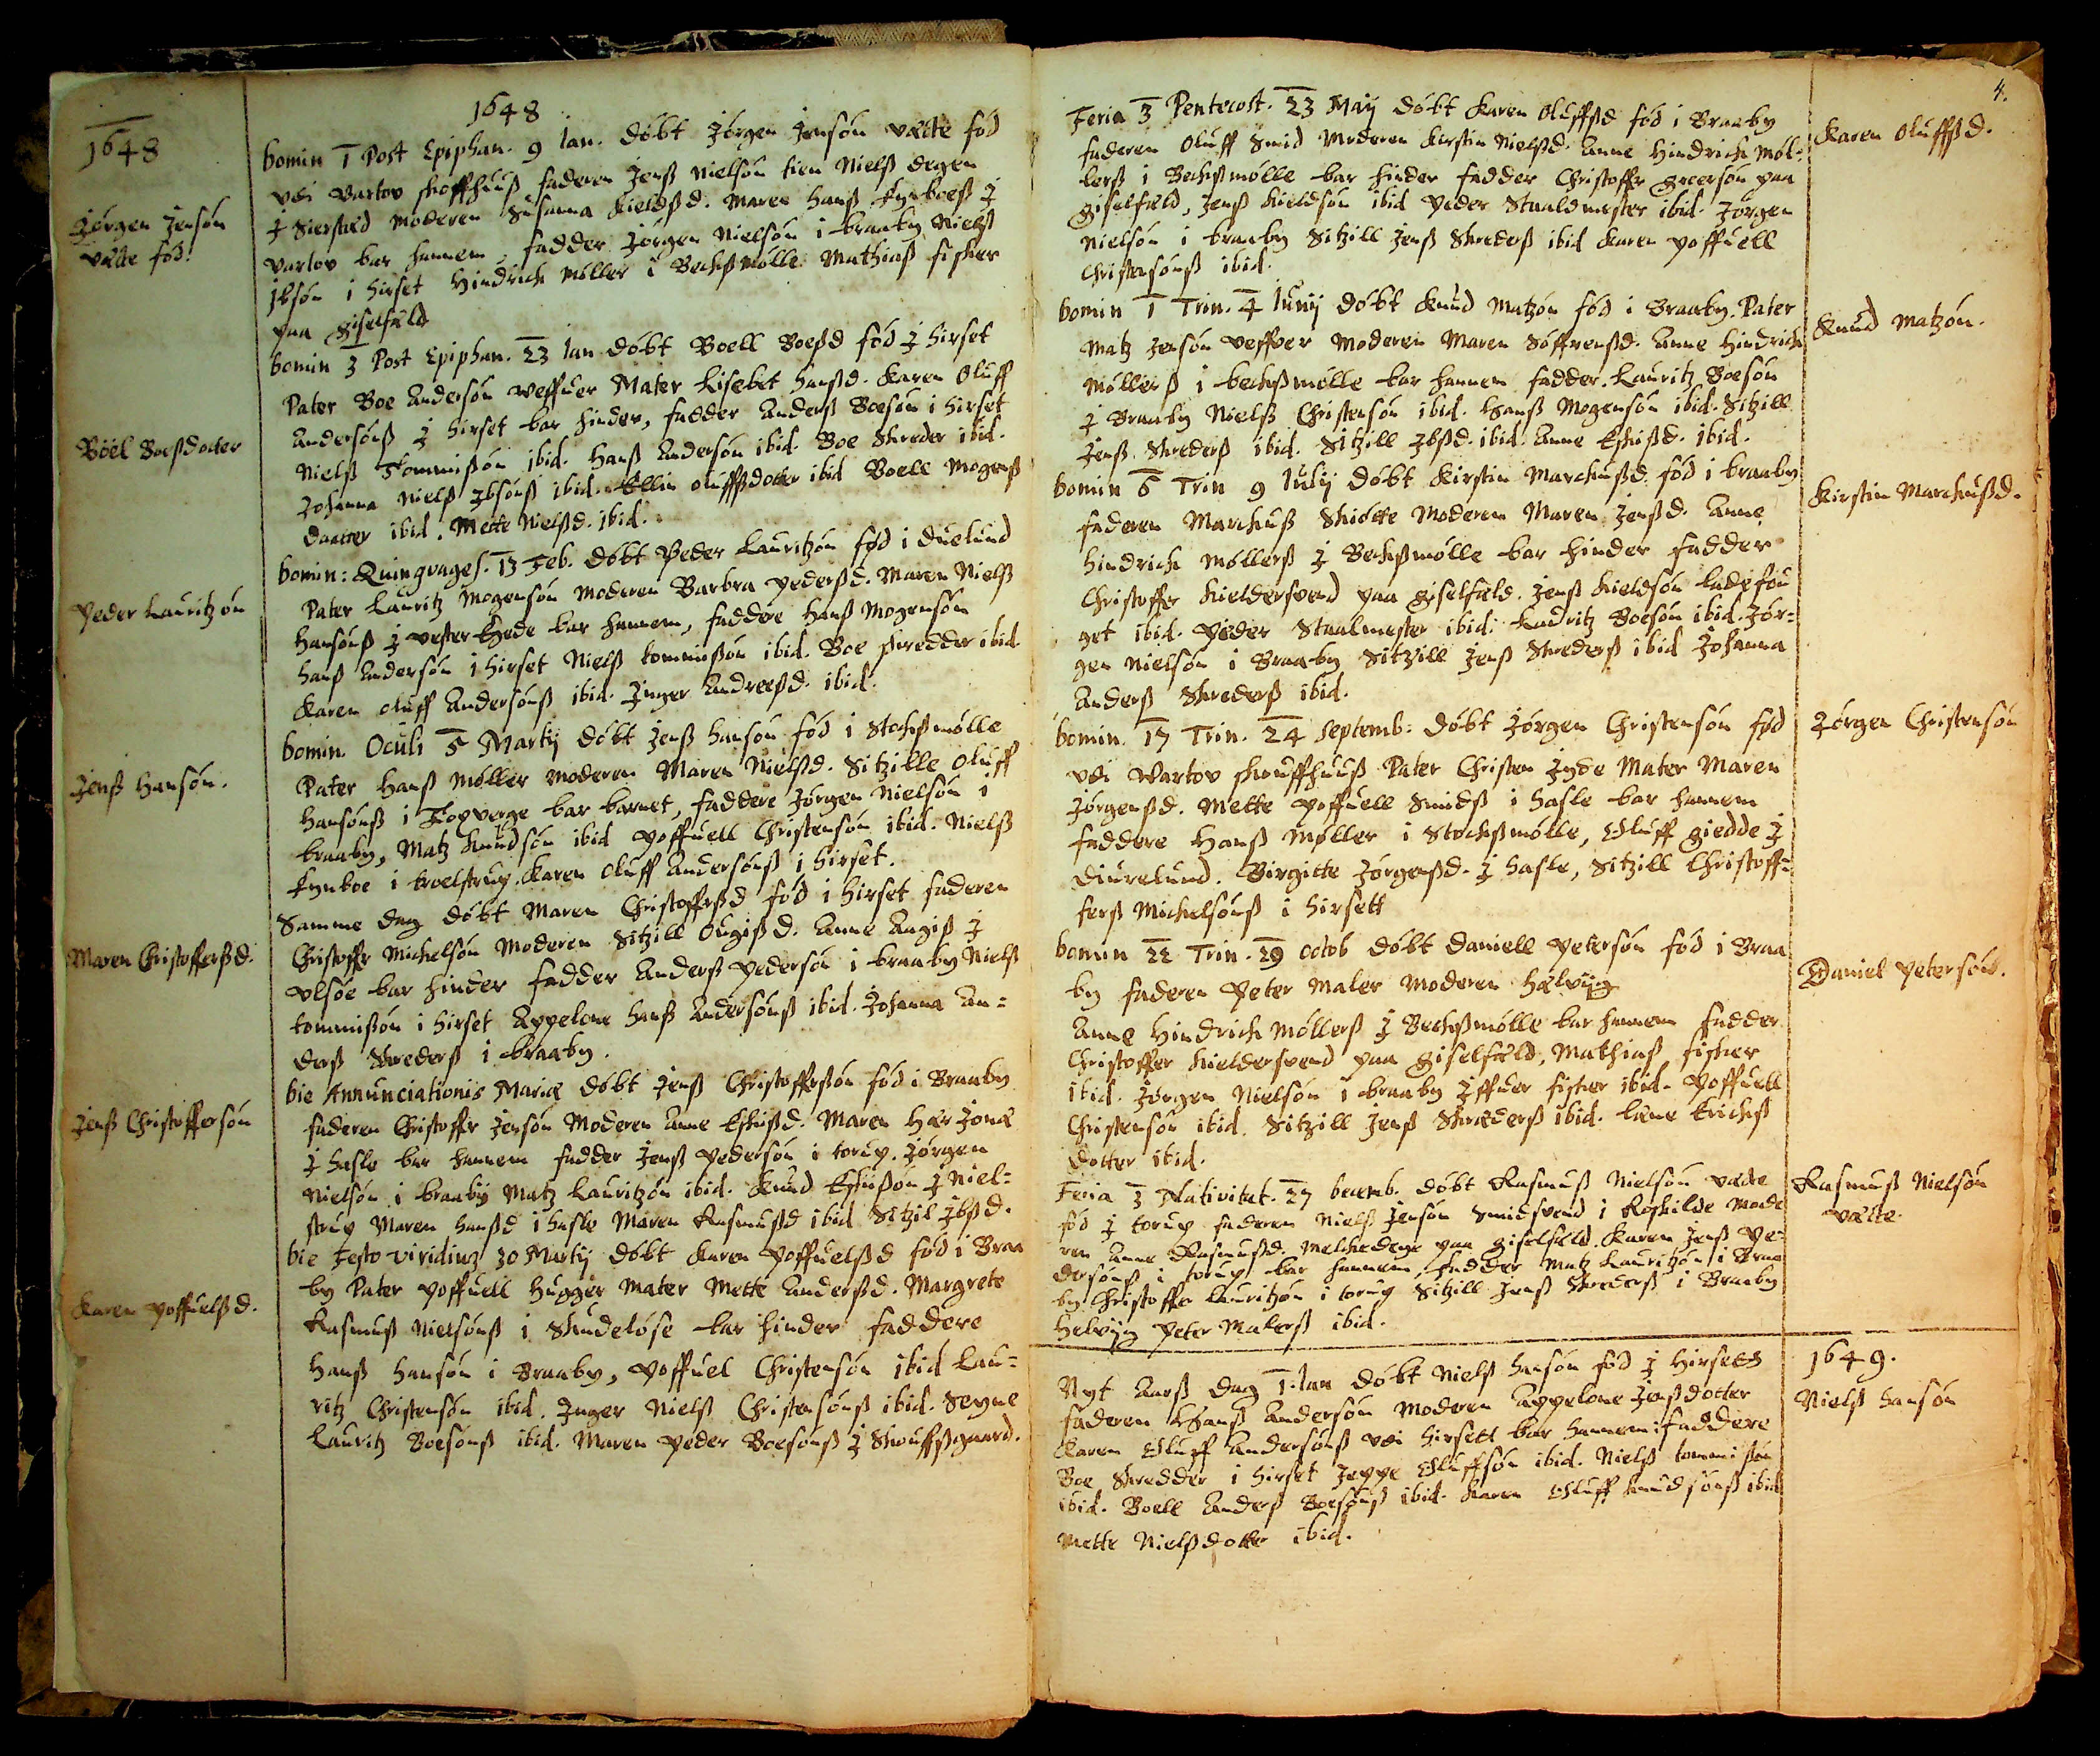1648
Jørgen Jensøn
uægte fød
1648
Domin 1 Post Epiphan. 9 Jan. døbt Jørgen Jensøn uecte fød
udi Vartov Skoffhuus Faderen Jens Nielsøn fra Niels Degen
J Sierstæd Moderen Susanna Kieldsd. Maren Hans Fynboes J
Vartov bar hannem, Fadder Jørgen Nielsøn i Braaby. Niels
Ibsøn i Hirset. Hindrick Møller i Becksmølle. Mathias Fisker
paa Giselfæld
Boel Boesdatter
Domin 3 Post Epiphan. 23 Jan. døbt Boell Boesd fød J Hirset
Pater Boe Andersøn Veffuer Mater Lisebet Hansd. Karen Oluff
Andersøns J Hirset bar hinder, Fadder Anders Boesøn i Hirset
Niels Tommisøn ibid. Hans Andersøn ibid. Boe Skræder ibid.
Johanna Niels Jbsøns ibid. Ellen## Oluffsdatter ibid. Boell Mogens
datter ibid. Mette Nielsd. ibid. 
Peder Lauritzøn 
Domin. Quinquages. 13 Feb. døbt Peder Lauritzøn fød i Duelund
Pater Lauritz Mogensøn Moderen Barbra Pedersd. Maren Niels
Hansøns J Vester Egede bar hannem, Faddere Hans Mogensøn
Hans Andersøn i Hirset Niels Tommisøn ibid. Boe Skredder ibid.
Karen Oluff Andersøns ibid. Inger Andreesd. ibid.
Jens Hansøn 
Domin Oculi 5. Marty døbt Jens Hansøn fød i Stochsmølle
Pater Hans Møller Moderen Maren Nielsd. Sitzille Oluff
Hansøns i Toxverge bar barnet, Faddere Jørgen Nielsøn i
Braaby, Matz Knudsøn ibid Poffuell Christensøn ibid. Niels
Fynboe i Troelstrup. Karen Oluff Andersøns i Hirset.
Maren Christoffersd. 
Samme dag. døbt Maren Christoffersd fød i Hirset. Faderen
Christoffer Mickelsøn Moderen Sitzill Ougisd. Anne Aagis J
Ulsøe bar hinder. Fadder Anders Pedersøn i Braaby. Niels
Tommisøn i Hirset Appelone Hans Andersøns ibid. Johanna An¬
ders Skreders i Braaby.
Jens Christoffersøn 
Die Annunciationis Mariæ døbt Jens Christoffersøn fød i Braaby
Faderen Christoffer Jensøn. Moderen Anne Eskisd. Maren Hær Jonæ
J Hasle bar hannem Fadder Jens Pedersøn i torup. Jørgen
Nielsen i Braaby. Matz Lauritzøn ibid. Knud Eskiisøn J Niel¬
strup. Maren Hansd. i Hasle. Marie Rasmusd. ibid. Sitzil Jbsd.
Karen Poffuelsdatter 
Die Festo Viridium 30. Marty døbt Karen Poffuelsd. fød i Braa
by Pater Poffuel Hugger Mater Mette Andersd. Margrete
Rasmus Nielsøns i Skudeløse bar hinder. Faddere 
Hans Hansøn i Braaby, Poffuel Christensøn ibid. Lau¬
ritz Christensøn ibid. Jnger Niels Christensøns ibid. Seigne
Lauritz Boesøns ibid. Maren Peder Boesøns J Skouffsgaard.
Feria 3 Pentecost. 23. May døbt Karen Oluffsd. fød i Braaby
Faderen Oluff Smid. Moderen Kirstin Nielsd. Anne Hindrick Møl¬
lers i Bechsmølle bar hinder Fadder Christoffer Greersøn paa
Giselfæld, Jens Kieldsøn ibid Peder Staaldmester ibid. Jørgen
Nielsøn i Braaby Sitzill Jens Skreders ibid Anne Poffuell
Christensøns ibid.
4.
Karen Oluffsd.
Domin 1 Trin. 4. Juny døbt Knud Matzøn fød i Braaby. Pater
Matz Jensøn Veffuer. Moderen Maren Søffrensd. Anne Hindrick
Møllers i Becks Mølle bar hannem. Fadder Lauritz Boesøn
J Braaby Niels Christensøn ibid. Hans Mogensøn ibid. Sitzill
Jens Skreders ibid. Sitzill Jbsd. ibid. Anne Eskisd. ibid.
Knud Matzøn
Domin 5 Trin. 9. July døbt Kirstin Marckusd. fød i Braaby
Faderen Marckus Skiøte. Moderen Maren Jensd. Anne
Hindrick Møllers J Becksmølle bar hinder Fadder
Christoffer Kieldersvend paa Giselfæld. Jens Kieldsøn ladefou
get ibid. Peder Staalmester ibid. Lauritz Boesøn ibid. Jør¬
gen Nielsøn i Braaby. Sitzill Jens Skreders ibid. Johanna
Anders Skræders ibid.
Kirstin Marckusdatter
Domin 17 Trin. 24. Septemb: døbt Jørgen Christensøn fød
udi Vartov Skouffhuus Pater Christen Jyde Mater Maren
Jørgensd. Mette Poffuell Smids i Hasle bar hannem
Faddere Hans Møller i Stocksmølle Oluff Giedde J
Diurelund. Birgitte Jørgensd. J Hasle. Sitzill Christoff¬
fers Mickelsøns i Hirsett
Jørgen Christensøn
Domin 22 Trin. 29. Octob døbt Daniell Petersøn fød i Braa
by. Faderen Peter Maler. Moderen Hælviig
Anne Hindrick Møllers J Becksmølle bar hannem Fadder
Christoffer Kieldersvend paa Giselfæld. Mathias Fisker
ibid. Jørgen Nielsøn i Braaby. Jffuer fisker ibid. Poffuel
Christensøn ibid. Sitzill Jens Skræders ibid. Læne Ericks
dotter ibid.
Daniel Petersøn
Feria 3 Nativitat 27. Decemb. døbt Rasmus Nielsøn Uæcte
fød J Torup Faderen Niels Jensøn Smidsvend i Roskilde. Mode
ren Anne Rasmusd. Melckedeye paa Giselfæld. Karen Jens Pe¬
dersøns i Torup bar hannem. Fadder Matz Lauritzøn i Braa
by. Christoffer Lauritzøn i torup. Sitzill Jens Skræders i Braaby.
Helvijg Peder Malers ibid.
Rasmus Nielsøn 
Uæcte
Nyt Aars Dag 1. Jan. døbt Niels Hansøn fød J Hirsett
Faderen Hans Andersøn. Moderen Appelone Jensdotter
Karen Oluff Andersøn udi Hirsett bar hannem: Faddere
Boe Skredder i Hirset. Jeppe Oluffsøn ibid. Niels Tommisøn
ibid. Boell Anders Boesøns ibid. Karen Oluff Knudsøns ibid
Mette Nielsdotter ibid. 
1649.
Niels Hansøn